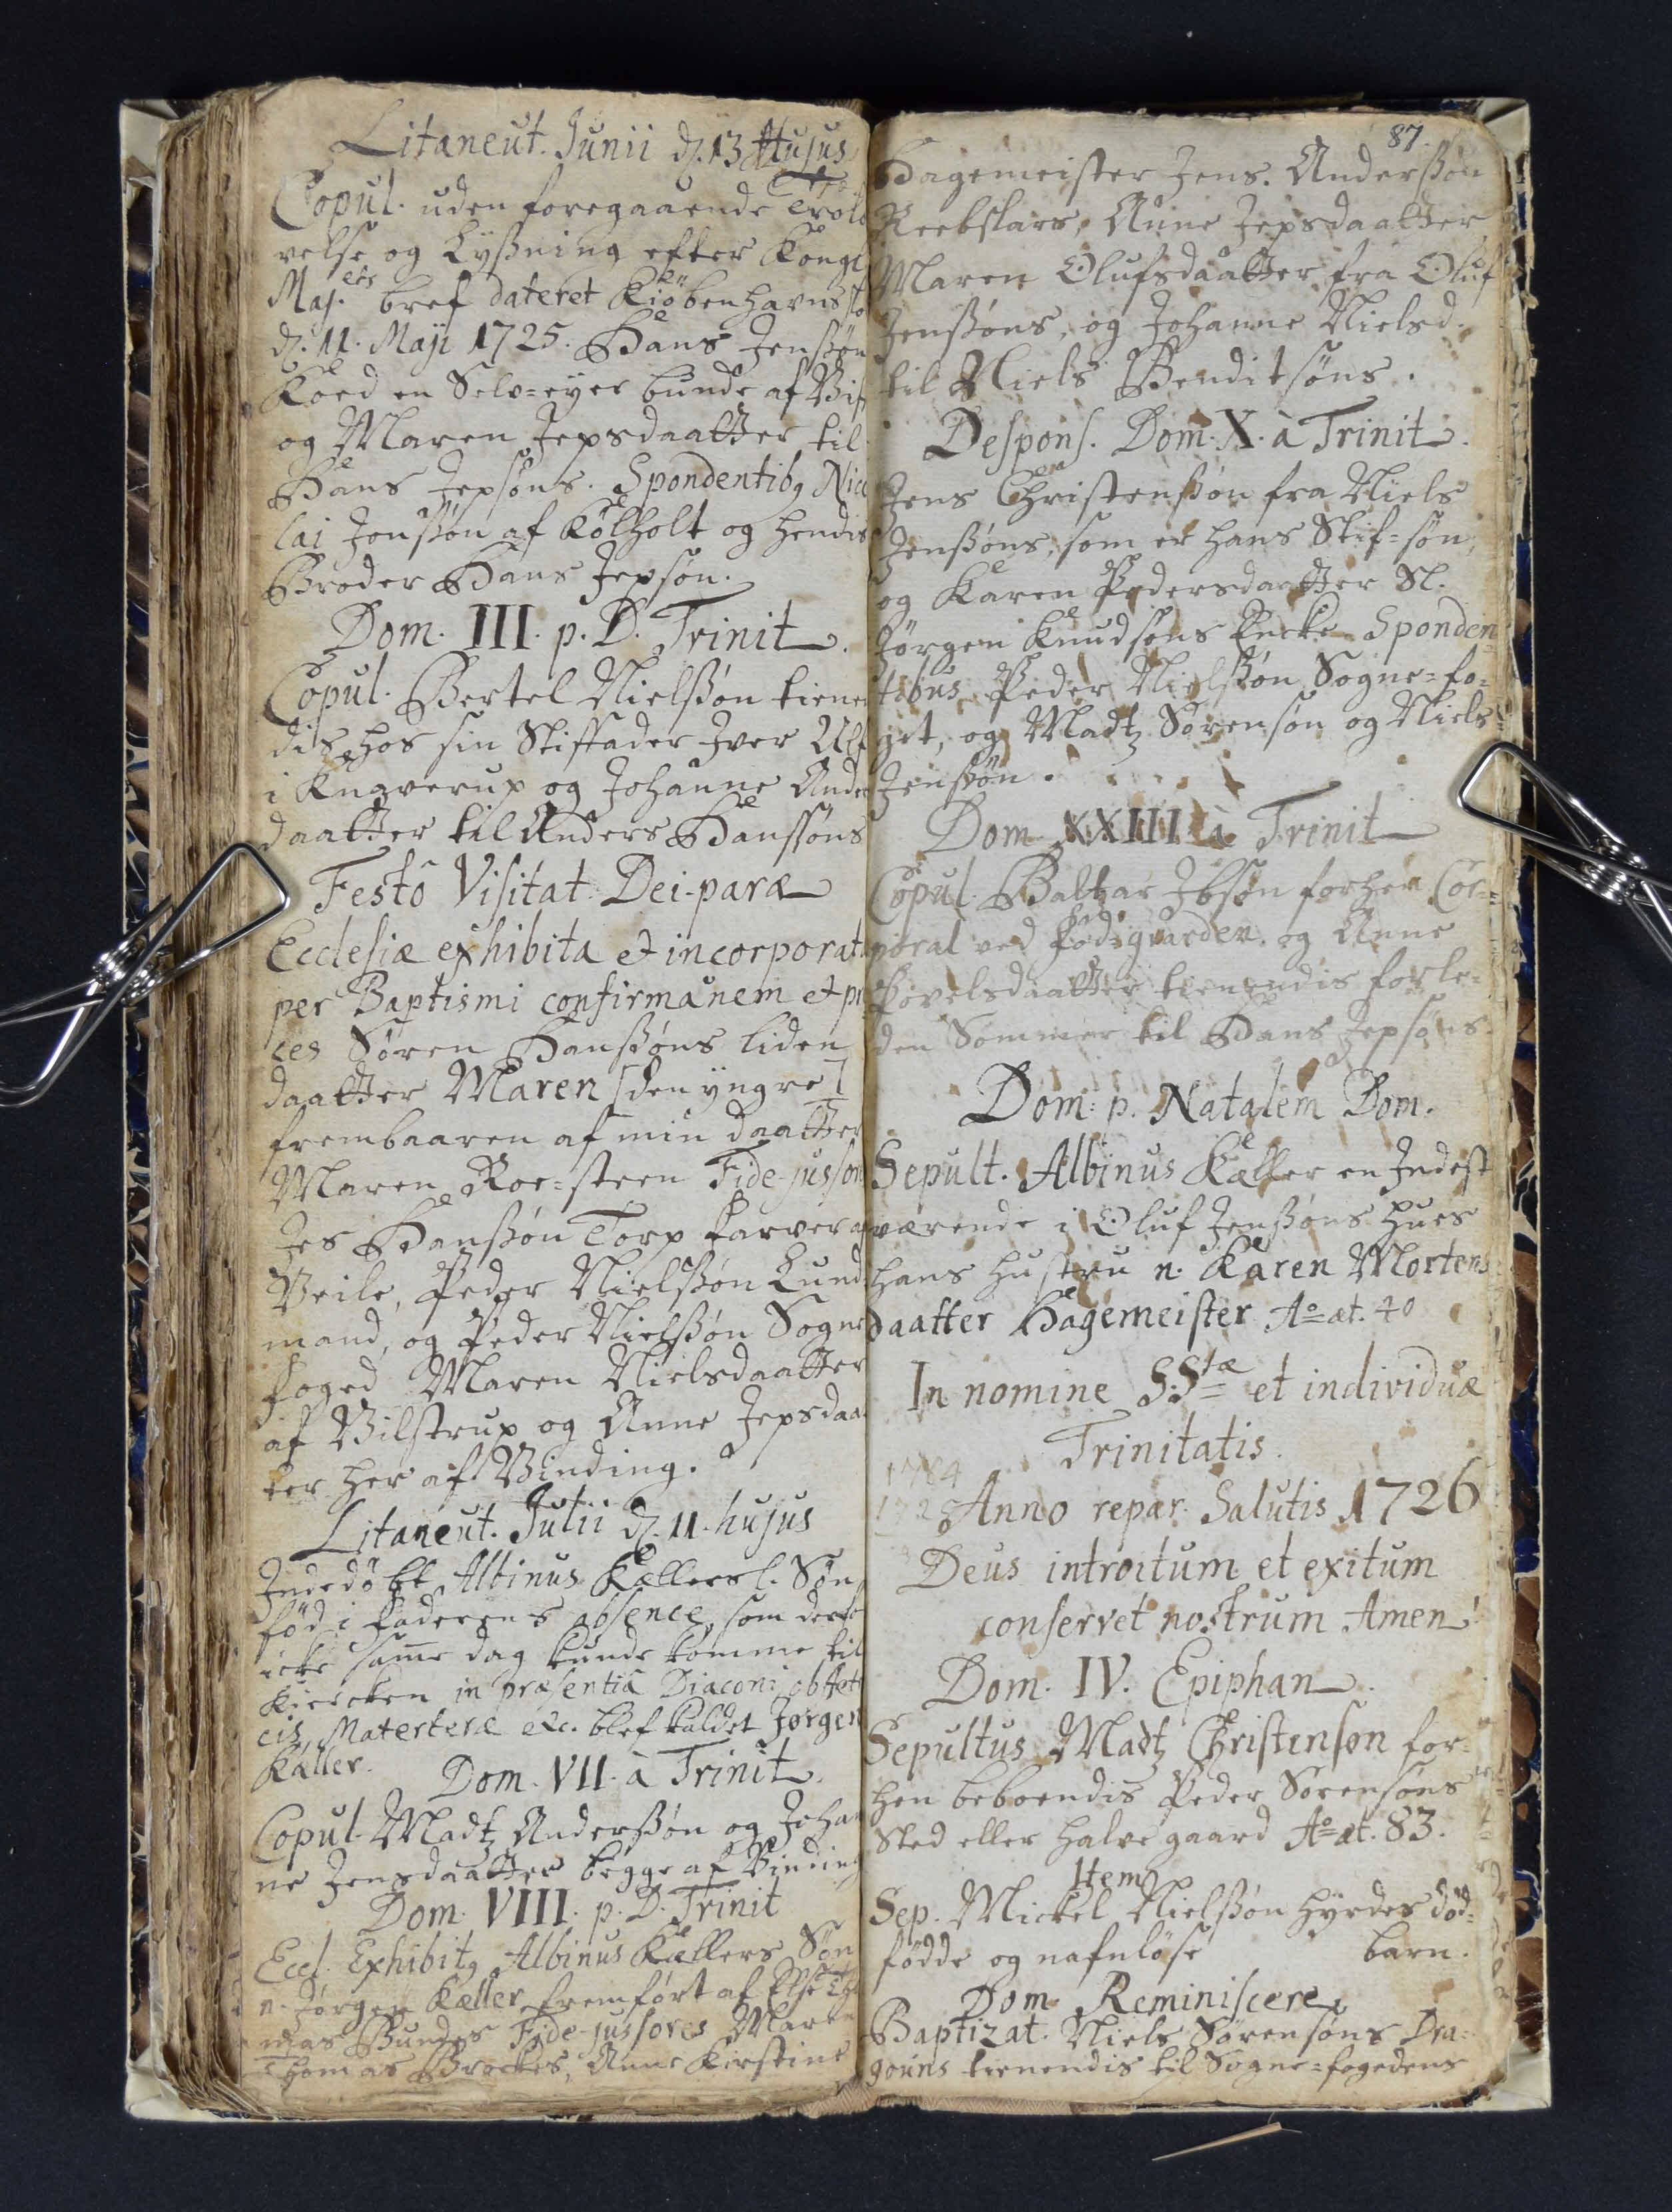Litaneut. Junii d. 13 Hujus
Copul. uden foregaaende Trolo
velse og Lysning efter Kongl
ets
Maj bref dateret Kiøbenhavns Slo 
d. 11. Maji 1725. Hans Jenssøn
Koed en Selv=eyer bunde af Vif 
og Maren Jepsdaatter til
Hans Jepsøns. Spondentibq Nico
lai Jonssøn af Kølholt og hendis
Broder Hans Jepsøn.
Dom. III. p. D. Trinit.
Copul. Bertel Nielssøn tienen
dis hos sin Stiffader Iver Ulf
i Knaverup og Johanne Ander 
daatter til Anders Hanssøns
Festo Visitat. Dei-paræ
Ecclesiæ exhibita et incorporat 
per Baptismi confirmanem et pr 
ces Søren Hanssøns liden
daatter Maren [den yngre]
frembaaren af min daatter
Maren Roe=steen Fide-jussor 
Jes Hanssøn Torp Farver af
Veile, Peder Nielssøn Lund
mand, og Peder Nielssøn Sogne
foged Maren Nielsdaatter
af Vilstrup og Anna Jepsdaa 
ter her af Vinding.
Litaneut. Julii d. 11. hujus
Indedøbt Albinus Kællers l. Søn
fød i faderens absense, som derfo 
icke same dag kunde komme til 
Kiercken in præsentia Diaconi obsteto
cis, Materteræ etc. blef kaldet Jørgen
Kæller.
Dom. VII. a Trinit.
Copul. Madtz Anderssøn og Johan
ne Jensdaatter, begge af Vinding
Dom. VIII. p. D. Trinit
Eccl; Exhibitq Albinus Kællers Søn
n. Jørgen Kæller, fremført af Else Th 
mas Bundes. Fide-jussores Maren
Thomas Brockes, Anne Kirstine
87.
Hagemeister Jens. Anderssøn
Reebslars, Anne Jepsdaatter
Maren Olufsdaatter fra Oluf
Jenssøns, og Johanne Nielsd.
til Niels Benditsøns
Despons. Dom. X. a Trinit.
Jens Christensøn fra Niels
Jenssøns, som er hans Stif=søn
og Karen Pedersdaatter Sl.
Jørgen Knudsøns Encke Sponden
tibus Peder Nielssøn Sogne=fo¬
get, og Madtz Sørensøn og Niels
Jenssøn.
Dom. XXIII. a Trinit
Copul. Baltzar Ibsøn forhen Cor¬
poral ved Fod=gvarden. og Anne
Povelsdaatter tienendis forle¬
den Sommer til Hans Jepsøns.
Dom: p. Natalem Dom.
Sepult. Albinus Kæller en Indest
værende i Oluf Jenssøns hues
hans hustru n. Karen Mortens
daatter Hagemeister Ao æt. 40
In nomine S.Stæ et individuæ
Trinitatis
Anno repær. Salutis1726
Deus introitum et exitum
conservet nostrum Amen
Dom. IV. Epiphan
Sepultus Madtz Christensøn for¬
hen beboendis Peder Sørensøns
Sted eller Halve gaard Ao æt. 83.
Item
Sep. Mickel Nielssøn Hyrdens død¬
fødde og nafnløse
barn.
Dom. Reminiscere
Baptizat. Niels Sørensøns Dra¬
gouns, tienendis til Sogne=fogdens
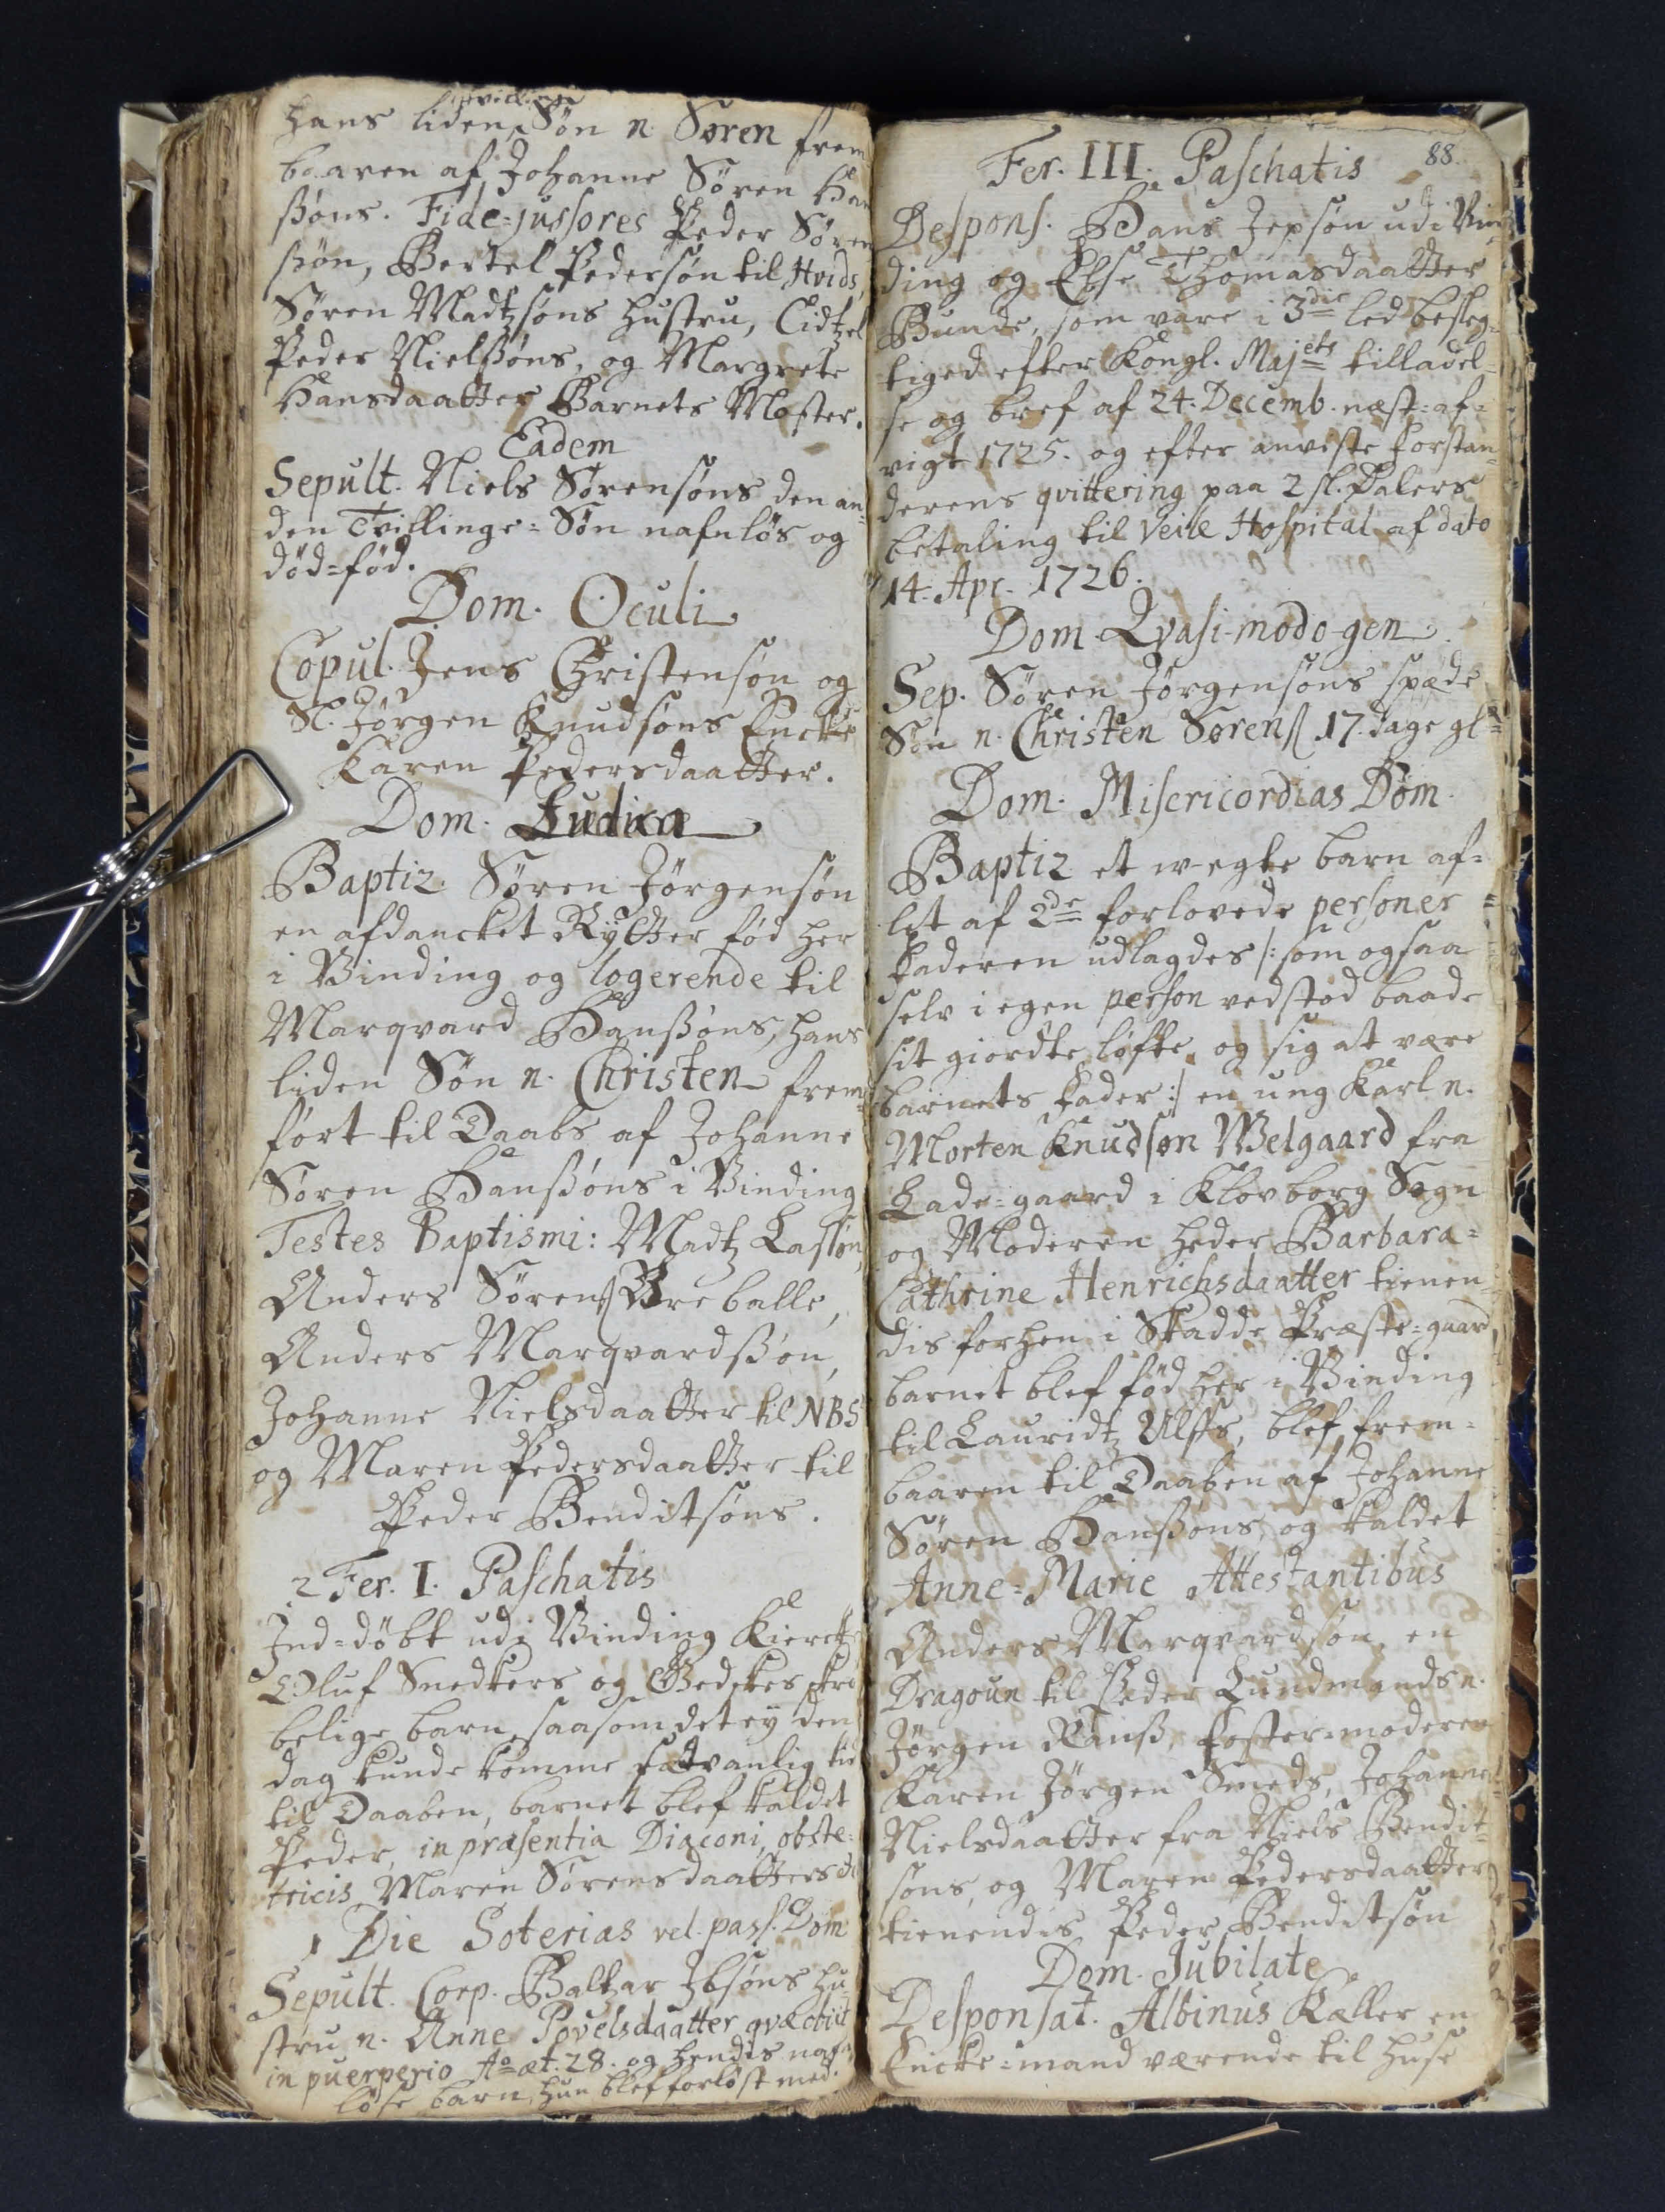##
hans liden Søn n. Søren, frem
baaren af Johanne Søren Han
ssøns. Fide-jussores Peder Søren
ssøn, Bertel Pedersøns til Hvids,
Søren Madtzsøns hustru, Cidtzel
Peder Nielssøns, og Margrete
Hansdaatter Barnets Moster.
Eadem
Sepult. Niels Sørensøns den an¬
den Tvillinge = Søn nafnløs og
død=fød.
Dom. Oculi
Copul. Jens Christensøn og
Sl. Jørgen Knudsøns Encke
Karen Pedersdaatter.
Dom. Judica
Baptiz. Søren Jørgensøn
en afdancket Rytter fød her
i Vinding og logerende til
Marqvard Hanssøns, hans
liden Søn n. Christen, frem¬
ført til Daabs af Johanne
Søren Hanssøns i Vinding
Testes Baptismi: Madtz Lassøn
Anders Sørens Breballe
Anders Marqvardssøn
Johanne Nielsdaatter til NBS
og Maren Pedersdaatter til
Peder Benditsøns
2 Fer. I. Paschatis
Ind=døbt udi Vinding Kiercke
Oluf Snedkers og Gedekes skrø
belige barn, saasom det ey den
dag kunde komme sædvanlig tid
til Daaben, baarnet blef kaldet
Peder, in prasentia Diaconi, obste¬
tricis Maren Sørensdaatter etc
Die Soterias vel. pa##. Dom
Sepult Corp. Baltzar Ibsøns hu
stru n. Anne Povelsdaatter qvæ obiit
in puerperio Ao æt. 28. og hendis nafn
løse barn hun blef forløst med
88.
Fer. III. Paschatis
Despons. Hans Jepsøn udi Vin
ding og Else Thomasdaatter
Bunde, som vare i 3die led besleg¬
tiged, efter Kongl. Majets tilladel¬
se og bref af 24. Decemb. næst=af¬
vigt 1725. og efter anviste Forstan¬
derens qvittering paa 2 sl. Dalers
betaling til Veile Hospital af dato
14. Apr. 1726.
Dom. Qvasi-modo-gen
Sep. Søren Jørgensøns spæde
Søn n. Christen Sørens 17 dage gl
Dom. Misericordias Dom
Baptiz et w-egte barn af¬
let af 2de forlovede personer
faderen udlagdes /: som ogsaa
selv i egen person vedstod baade
sit giordte løfte, og sig at være
barnets fader :/ en ung Karl n.
Morten Knudsøn Welgaard fra
Lade=gaard i Klovborg Sogn
og Moderen heder Barbara =
Cathrine Henrichsdaatter tienen¬
dis forhen i Skadde Præste=gaard
barnet blef fød her i Vinding
til Lauridtz Ulffs, blef frem¬
baaren til Daaben af Johanne
Søren Hanssøns, og kaldet
Anne=Marie Attestantibus
Anders Marqvardsøn. en
Dragoun til Peder Lundmands n.
Jørgen Ranss, Foster=moderen
Karen Jørgen Smeds, Johanne
Nielsdaatter fra Niels Bendit¬
søns, og Maren Pedersdaatter
tienendis Peder Benditsøn
Dom. Jubilate
Desponsat. Albinus Kæller en 
Encke=mand værende til huse